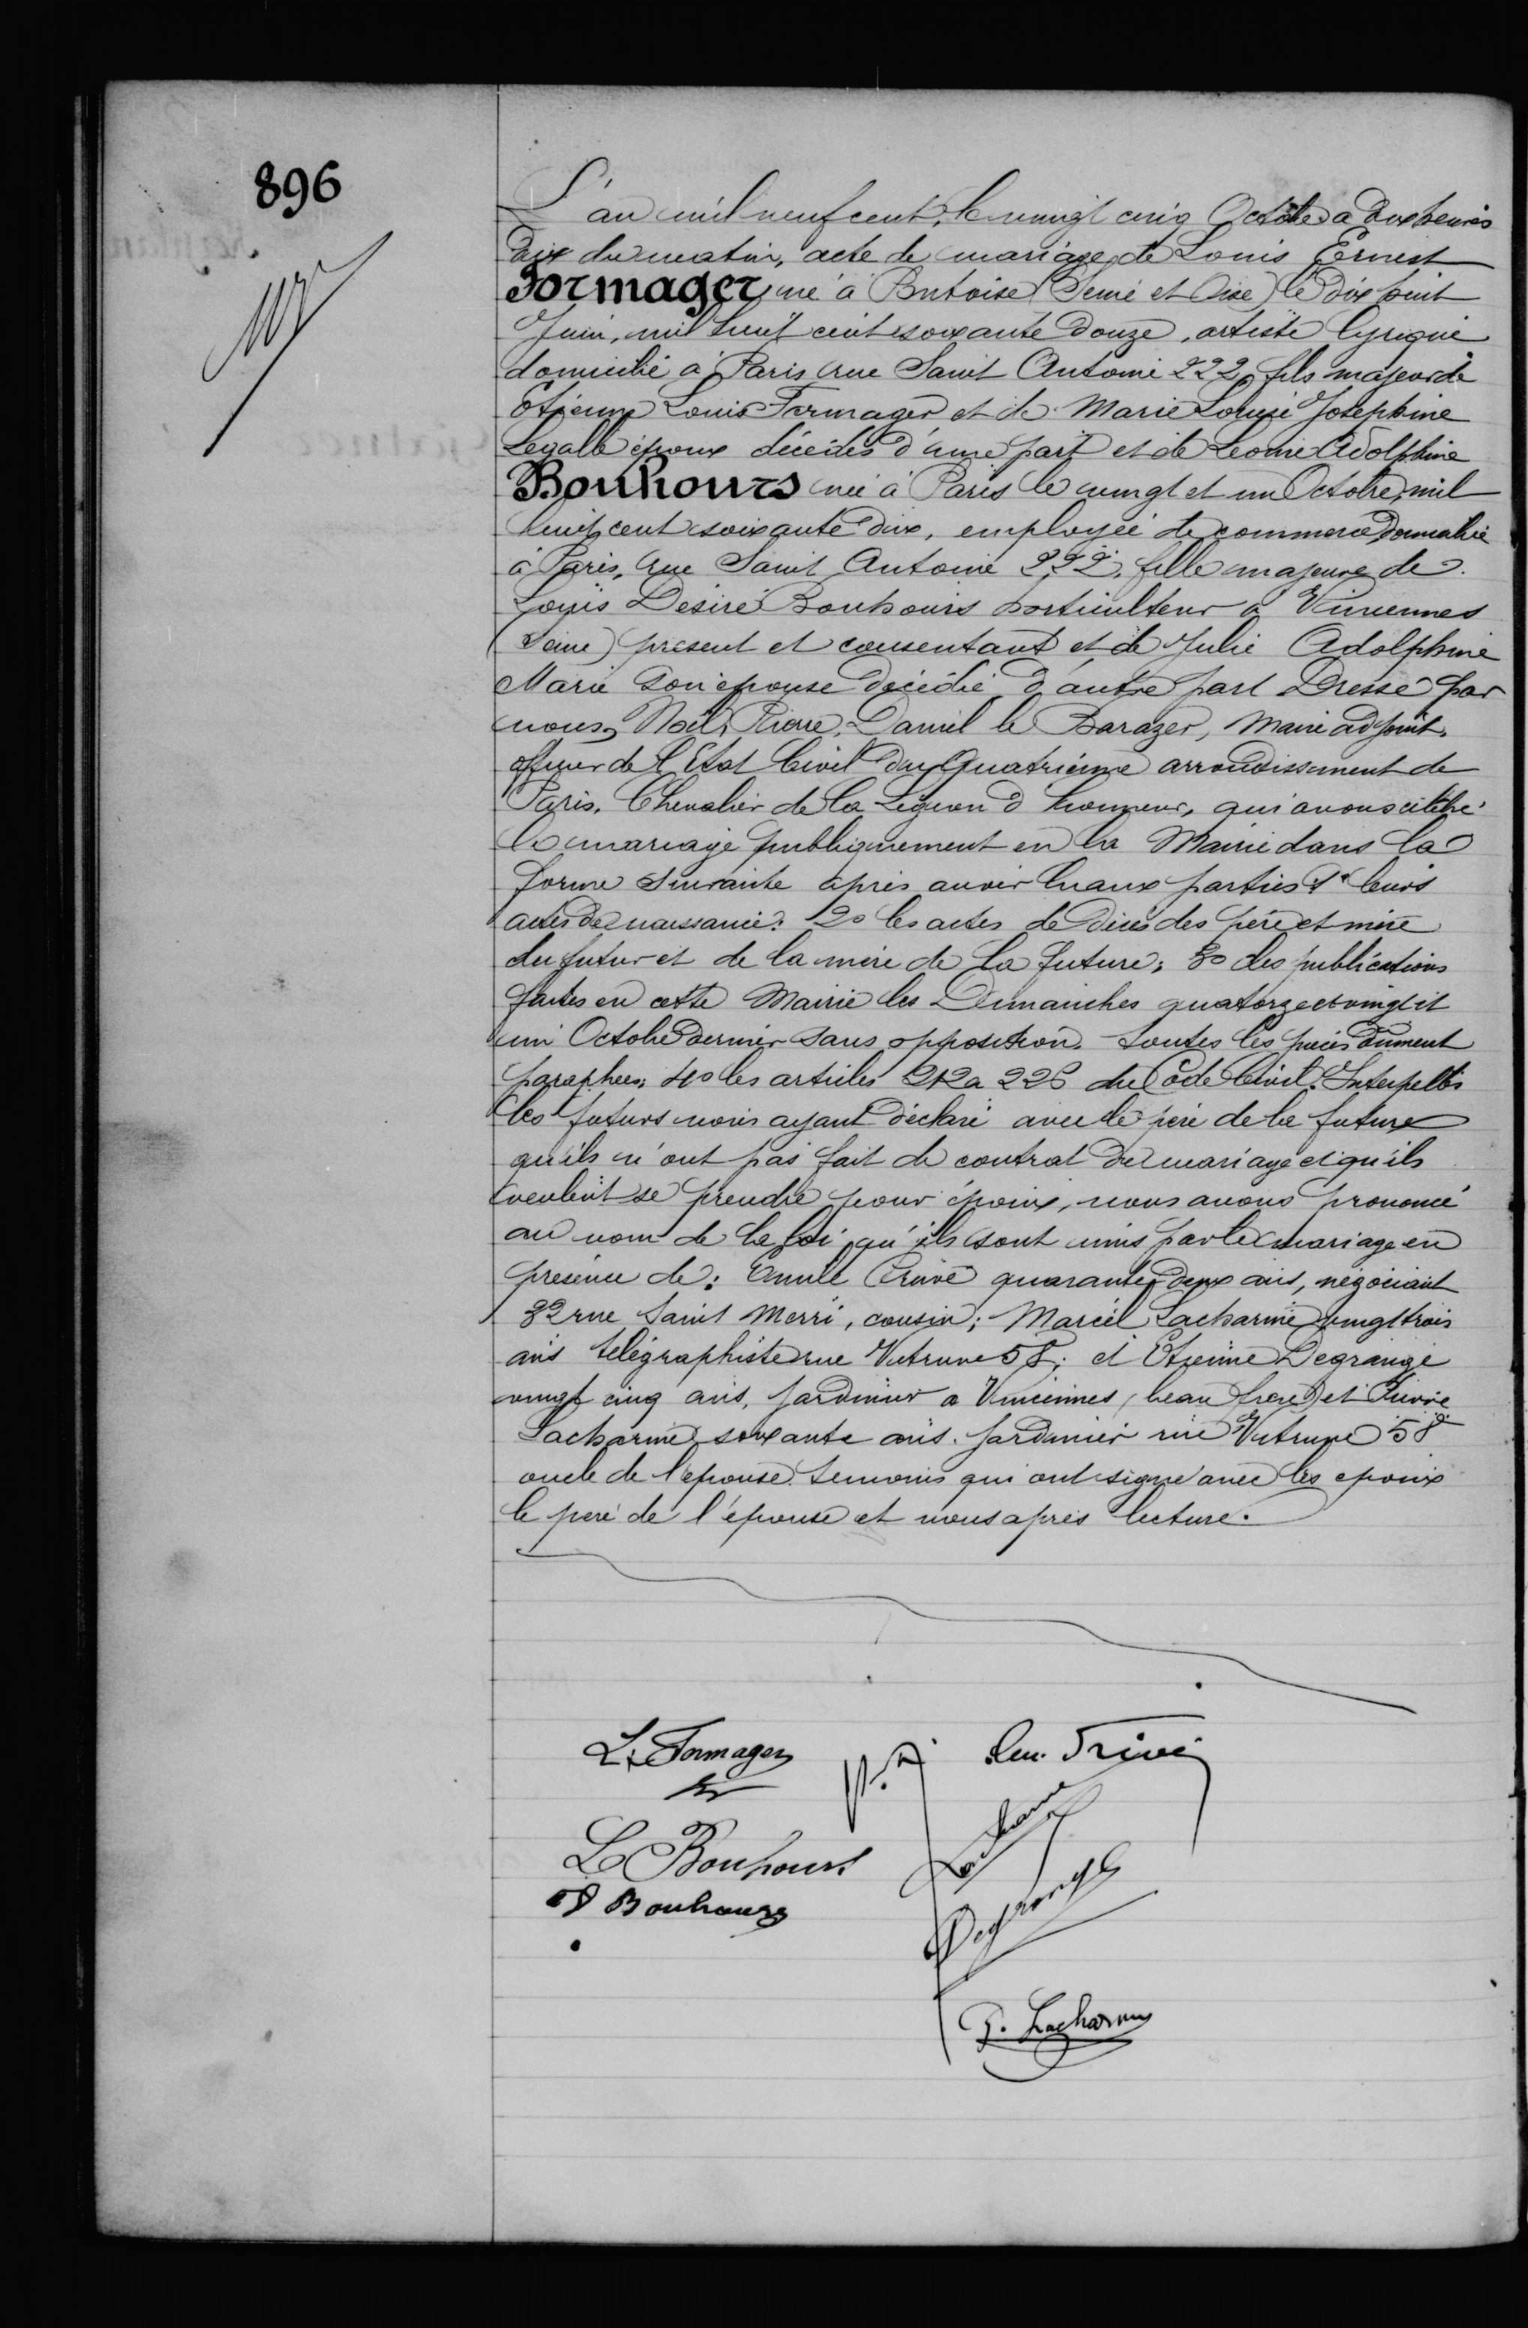L'an mil neuf cent le vingt cinq Octobre a dix heures
dix du matin, acte de mariage de Louis Ernest
Formager, né à Butoise (Seine et Oise) le dix huit
Juin mil huit cent soixante douze, artiste lyrique
domicilié à Paris rue Saint Atoine 222 fils majeur de
Etienne Louis Formager et de Marie Louise Joséphine
Legalle époux décédés d'une part et de Léonie Adolphine
Bouthours née à Paris le vingt et un Octobre mil
huit cent soixante dix, employée de commerce domicilié
à Paris, rue Saint Antoine 222, fille majeure de
Louis Désiré Boubours horticulteur à Vincennes
(Seine) présent et consentant et de Julie Adolphine
Marie son épouse décédée d'autre part Dressé par
nous, Noël Pierre Daniel le Barager, maire adjoint
officier de l'Etat Civil du quatrième arrondissement de
Paris, Chevalier de la Légion d'honneur qui avons célébré
le mariage publiquement en la Mairie dans la
forme suivante après avoir lu aux parties 1° leurs
actes de naissance: 2° les actes de décès des père et mère
du futur et de la mère de la future, 3° les publications
faites en cette mairie les Dimanches quatorze et vingt et
un Octobre dernier sans opposition toutes les pièces dûment
paraphées, 4° les articles 212 a 226 du Code Civil. Interpellés
les futurs nous ayant déclaré avec le père de la future
qu'ils n'ont pas fait de contrat de mariage et qu'ils
veulent se prendre pour époux, nous avons prononcé
au nom de la loi qu'ils sont unis par le mariage en
présence de: Emile Prave quarante deux ans, négociant
32 rue Saint Merri, cousin; Marcel Lacharme vingt trois
ans télégraphiste rue Vitrune 58; et Etienne Degrange
vingt cinq ans, jardinier a Vincennes, beau frère et Anne
Lacharme soixante ans, jardinier rue Vitrune 58
oncle de l'epouse témoins qui ont signé avec les epoux
le père de l'epouse et nous après lecture.
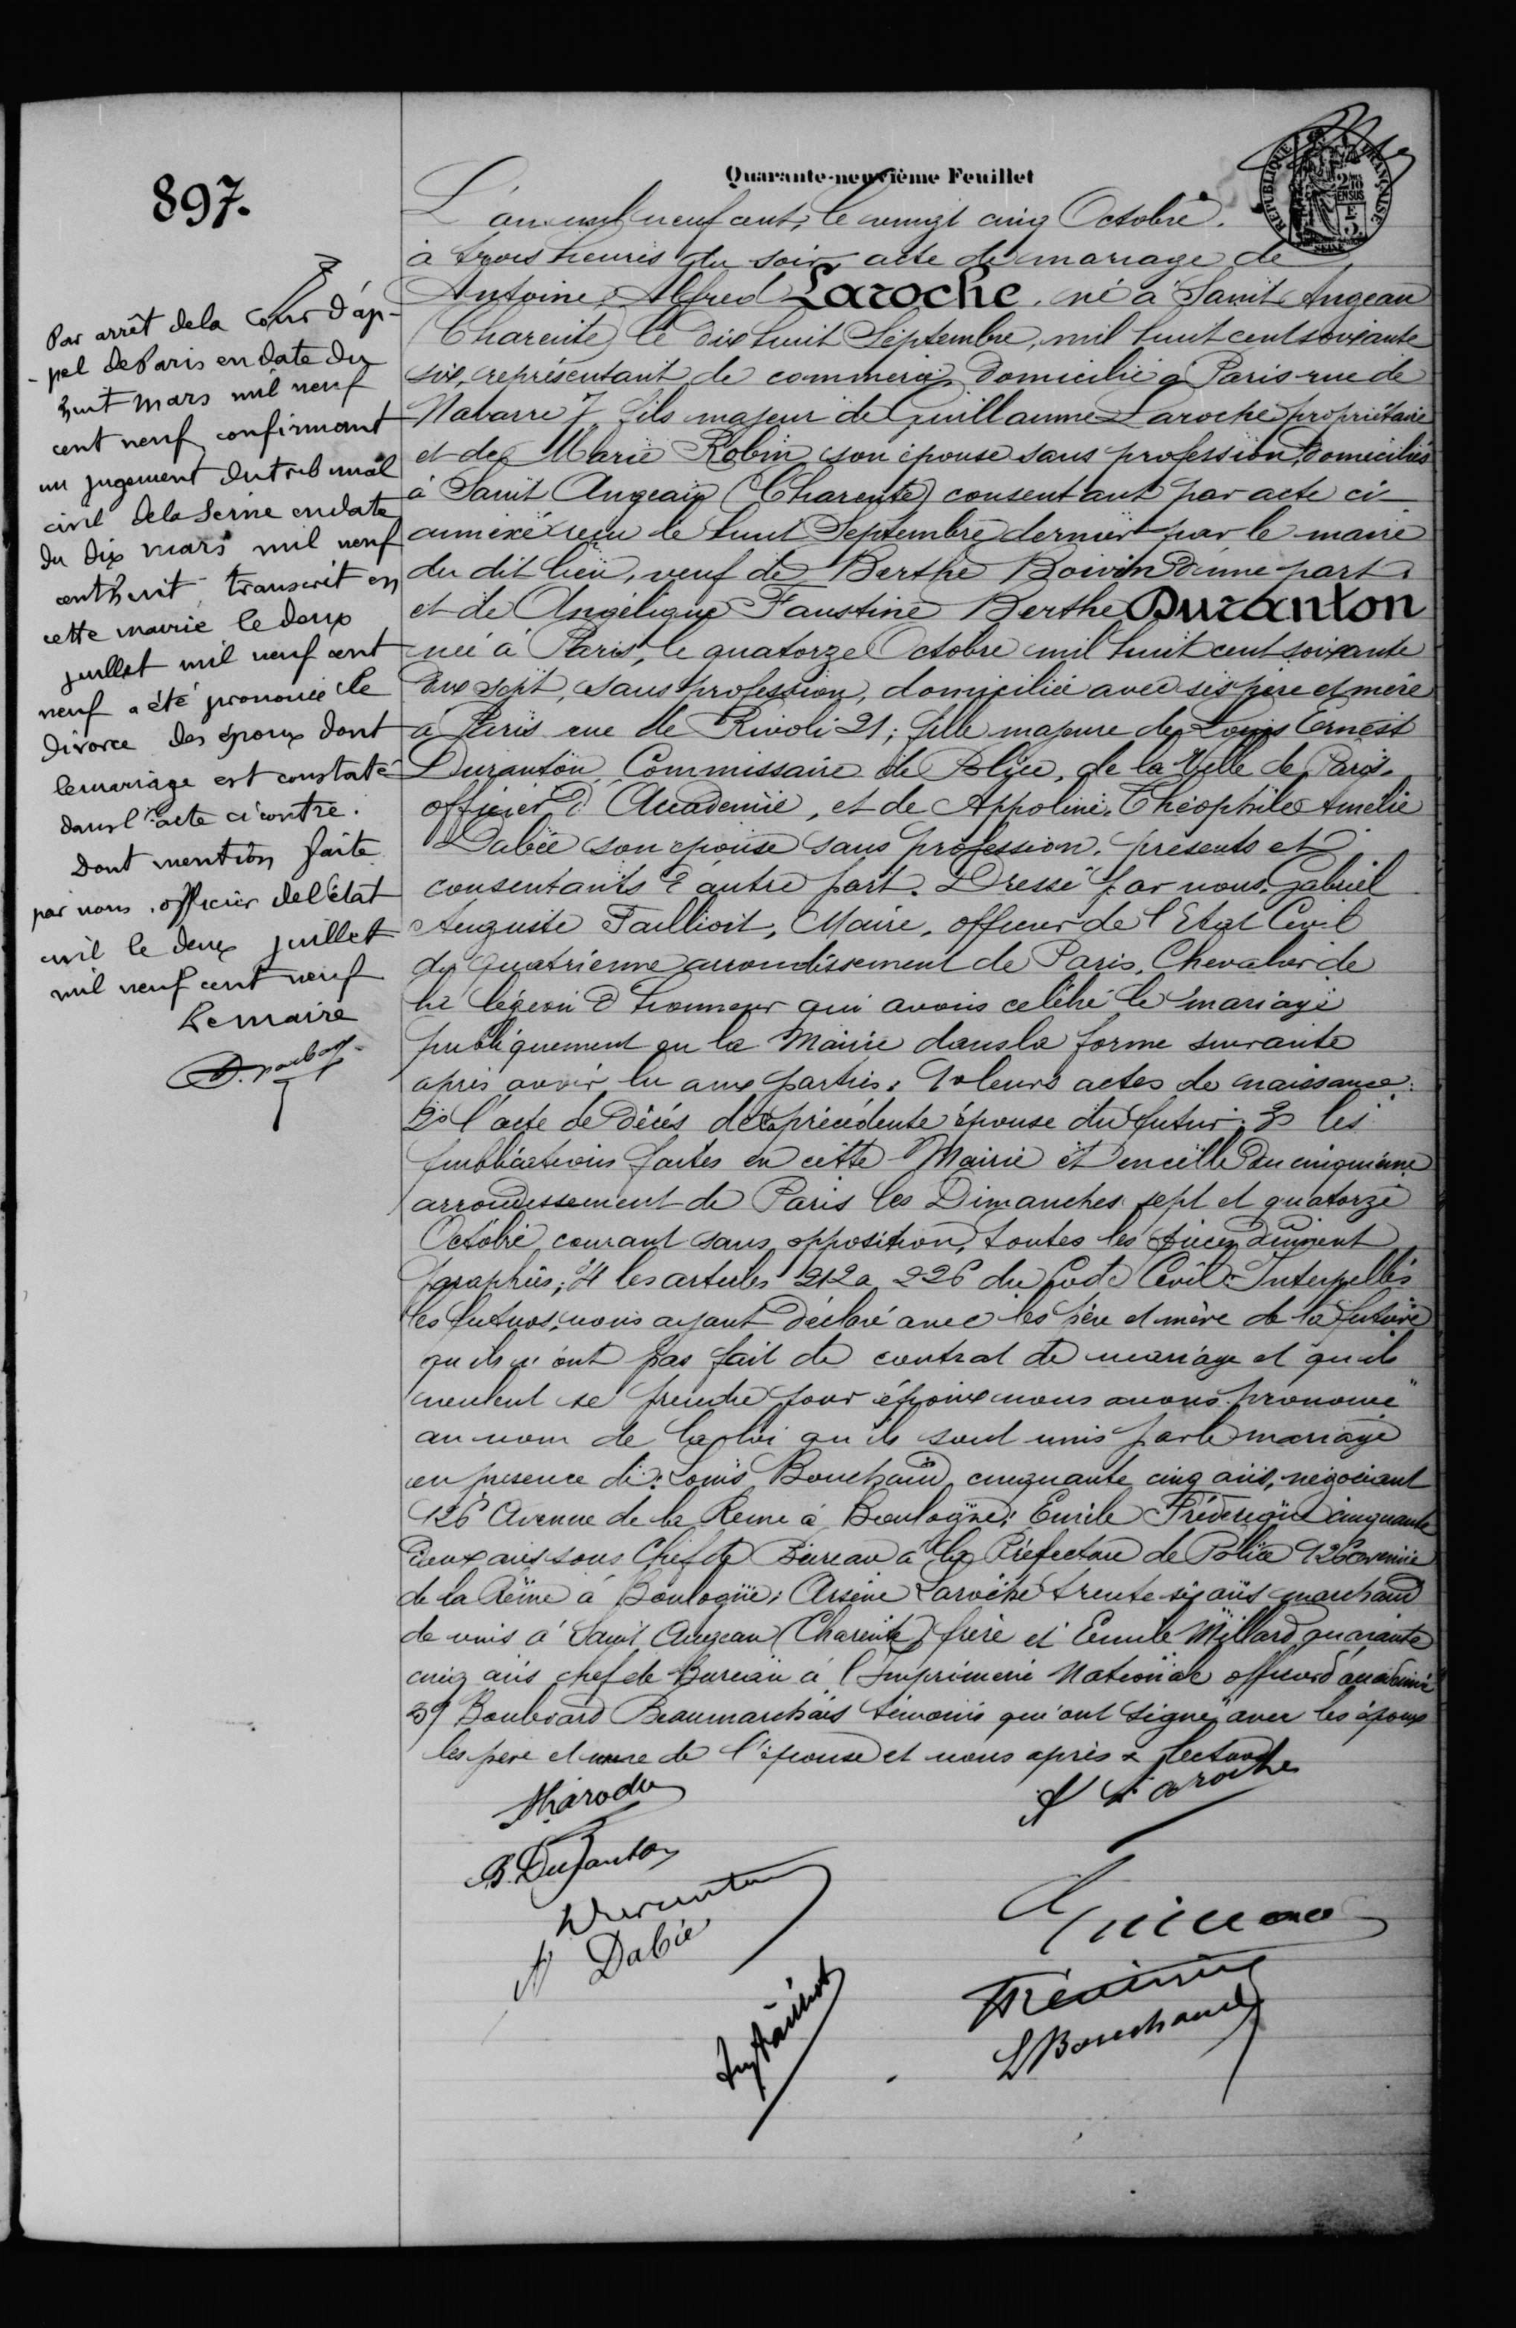L'an mil neuf cent, le vingt cinq Octobre
à trois heures du soir acte de mariage de
Antoine Alfred Laroche, né à Saint Anceau
(Charente) le dix huit Septembre, mil huit cent soixante
six, représentant de commerce domicilié à Paris rue de
Navarre 7 fils majeur de Guillaume Laroche propriétaire
et de Marie Robin son épouse sans profession, domiciliés
à Saint Angeais (Charente) consentant par acte ci-
annexé reçu le huit Septembre dernier par le maire
du dit lieu, veuf de Berthe Boivin d'une part.
et de Angélique Faustine Berthe Duranton
née à Paris, le quatorze Octobre mil huit cent soixante
dix sept, sans profession, domiciliée avec ses père et mère
à Paris rue de Rivoli 21; fille majeure de Louis Ernest
Duranton, Commissaire de Police de la Ville de Paris,
officier d'Académie, et de Appoline Théophile Amélie
Dabée son épouse sans profession présents et
consentants d'autre part. Dressé par nous Gabriel
Auguste Faillioit, Maire, officier de l'Etat Civil
du quatrième arrondissement de Paris, Chevalier de
la Légion d'Honneur qui avons célébré le mariage
publiquement en la mairie dans la forme suivante
après avoir lu aux parties: 1° leurs actes de naissance
2° l'acte de décès de la précédente épouse du futur; 3° les
publications faites en cette Mairie et en celle du cinquième
arrondissement de Paris les Dimanche sept et quatorze
Octobre courant sans opposition, toutes les pièces dument
paraphées; 4° les articles 212 a 226 du Code Civil: Interpellés
les futurs nous ayant déclaré avec les père et mère de la future
qu'ils n'ont pas fait de contrat de mariage et qu'ils
veulent se prendre pour époux nous avons prononcé
au nom de la loi qu'ils sont unis par le mariage
en présence de: Louis Bouchain cinquante cinq ans, négociant
126 Avenue de la Reine à Boulogne; Emile Frédérique cinquante
deux ans sous chef de bureau de la Préfecture de Police 126 avenue
de la Reine à Boulogne; Arsène Laroche trente six ans marchand
de vins à Saint Auzeau (Charente) frère d'Ernest Millard, quarante
cinq ans chef de Bureau de l'Imprimerie national officier d'académie
39 Boulevard Beaumarchais témoins qui ont signé avec les époux
les père et mère de l'épouse et nous après lecture